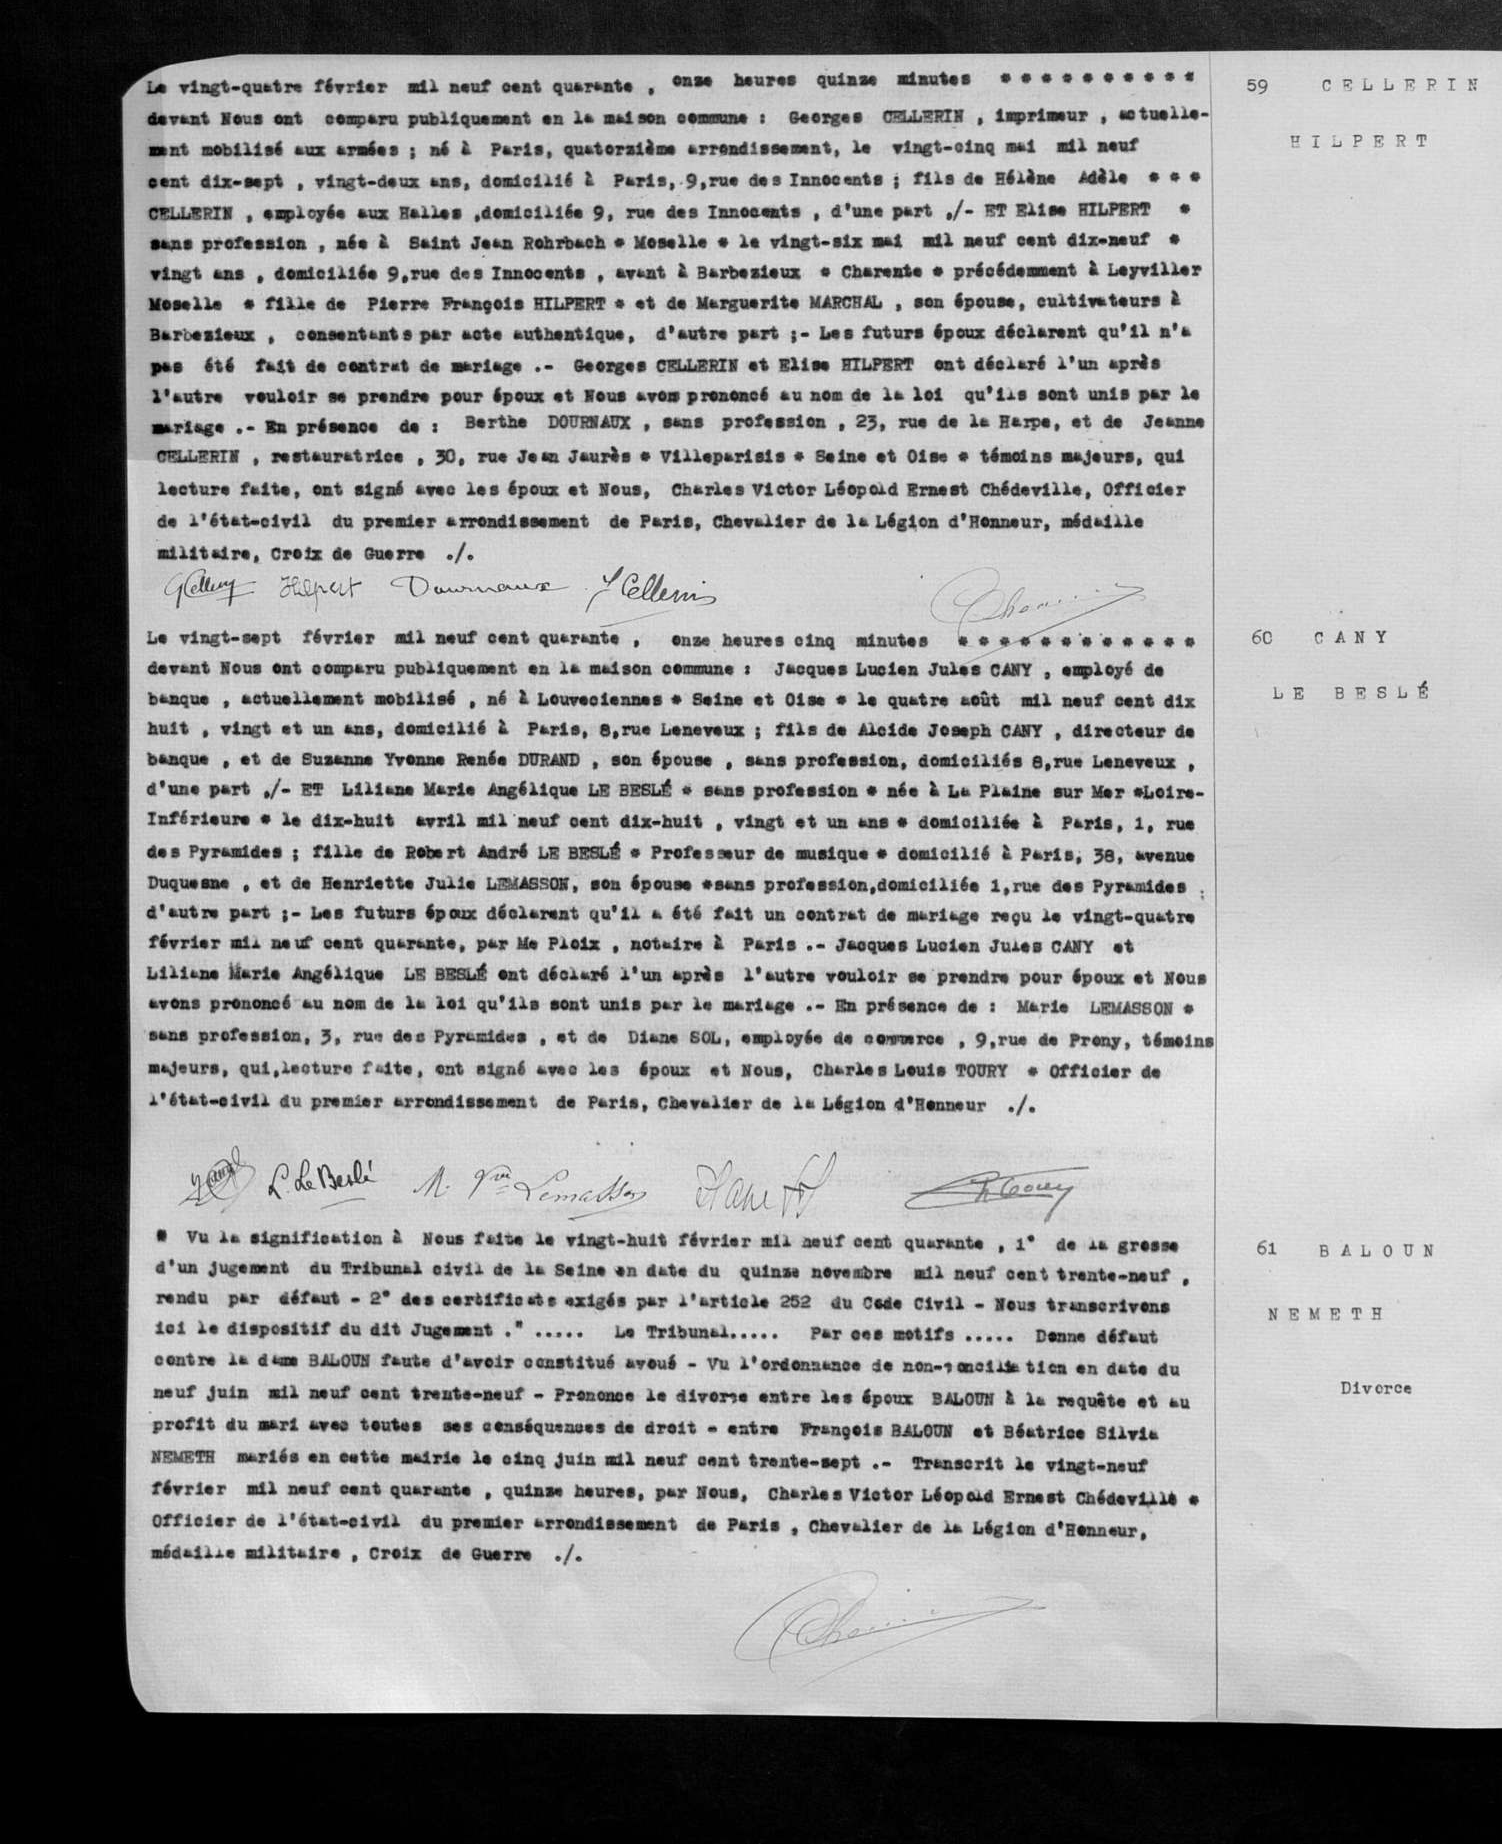Le vingt-quatre février mil neuf cent quarante, onze heures quinze minutes ****
devant Nous ont comparu publiquement en la maison commune: Georges CELLERIN, imprimeur, actuelle-
ment mobilisé aux armées; né à Paris, quatorzième arrondissement, le vingt-cinq mai mil neuf
cent dix-sept, vingt-deux ans, domicilié à Paris, 9, rue des Innocents; fils de Hélène Adèle ***
CELLERIN, employée aux Halles, domiciliée 9, rue des Innocents, d'une part ,/-ET Elise HILPERT *
sans profession, née à Saint Jean Rohrbach * Moselle * le vingt-six mai mil neuf cent dix-neuf *
vingt ans, domiciliée 9, rue des Innocents, avant à Barbezieux * Charente * précédemment à Leyviller
Moselle * fille de Pierre François HILPERT * et de Marguerite MARCHAL, son épouse, cultivateurs à
Barbesieux, consentants par acte authentique, d'autre part ;-Les futurs époux déclarent qu'il n'a
pas été fait de contrat de mariage .-George CELLERIN et Elise HILPERT ont déclaré l'un après
l'autre vouloir se prendre pour époux et Nous avons prononcé au nom de la loi qu'ils sont unis par le
mariage .-En présence de: Berthe DOURNAUX, sans profession, 23, rue de la Harpe, et de Jeanne
CELLERIN, restauratrice, 30, rue Jean Jaurès * Villeparisis * Seine et Oise * témoins majeurs, qui
lecture faite, ont signé avec les époux et Nous, Charles Victor Léopold Ernest Chédeville, Officier
de l'état-civil du premier arrondissement de Paris, Chevalier de la Légion d'Honneur, médaille
militaire, Croix de Guerre ./.
Le vingt-sept février mil neuf cent quarante, onze heures cinq minutes ****
devant Nous ont comparu publiquement en la maison commune: Jacques Lucien Jules CANY, employé de
banque, actuellement mobilisé, né à Louveciennes * Seine et Oise * le quatre août mil neuf cent dix
huit, vingt et un ans, domicilié à Paris, 8, rue Leneveux; fils de Alcide Joseph CANY, directeur de
banque, et de Suzanne Yvonne Renée DURAND, son épouse, sans professions, domiciliés 8, rue Leneveux,
d'une part ,/-ET Liliane Marie Angélique LE BESLE * sans profession * née à La Plaine sur Mer * Loire-
Inférieure * le dix-huit avril mil neuf cent dix-huit, vingt et un ans * domiciliée à Paris, 1, rue
des Pyramides; fille de Robert André LE BESLE * Professeur de musique * domicilié à Paris, 38, avenue
Duquesne, et de Henriette Julie LEMASSON, son épouse * sans profession, domiciliée 1, rue des Pyramides
d'autre part ;-Les futurs époux déclarent qu'il a été fait un contrat de mariage reçu le vingt-quatre
février mil neuf cent quarante, par Me Ploix, notaire à Paris .-Jacques Lucien Jules CANY et
Liliane Marie Angélique LE BESLE ont déclaré l'un après l'autre vouloir se prendre pou époux et Nous
avons prononcé au nom de la loi qu'ils sont unis par le mariage .-En présence de: Marie LEMASSON *
sans profession, 3, rue des Pyramides, et de Diane SOL, employée de commerce, 9, rue de Prony, témoins
majeurs, qui, lecture faite, ont signé avec les époux et Nous, Charles Louis TOURY * Officier de
l'état-civil du premier arrondissement de Paris, Chevalier de la Légion d'Honneur ./.
Vu la signification à Nous faite le vingt-huit février mil neuf cent quarante, 1° de la grosse
d'un jugement du Tribunal civil de la Seine en date du quinze novembre mil neuf cent trente-neuf,
rendu par défaut-2° des certificats exigés par l'article 252 du Code Civil-Nous transcrivons
ici le dispositif du dit Jugement ." .... Le Tribunal.... Par ces motifs..... Donne défaut
contre la dame BALOUN faute d'avoir constitué avoué-Vu l'ordonnance de non-conciliation en date du
neuf juin mil neuf cent trente-neuf-Prononce le divorce entre les époux BALOUN à la requête et au
profit du mari avec toutes ses conséquences de droit-entre François BALOUN et Béatrice Silvia
NEMETH mariés en cette mairie le cinq juin mil neuf cent trente-sept .-Transcrit le vingt-neuf
février mil neuf cent quarante, quinze heures, par Nous, Charles Victor Léopold Ernest Chédeville *
Officier de l'état-civil du premier arrondissement de Paris, Chevalier de la Légion d'Honneur,
médaille militaire, Croix de Guerre ./.
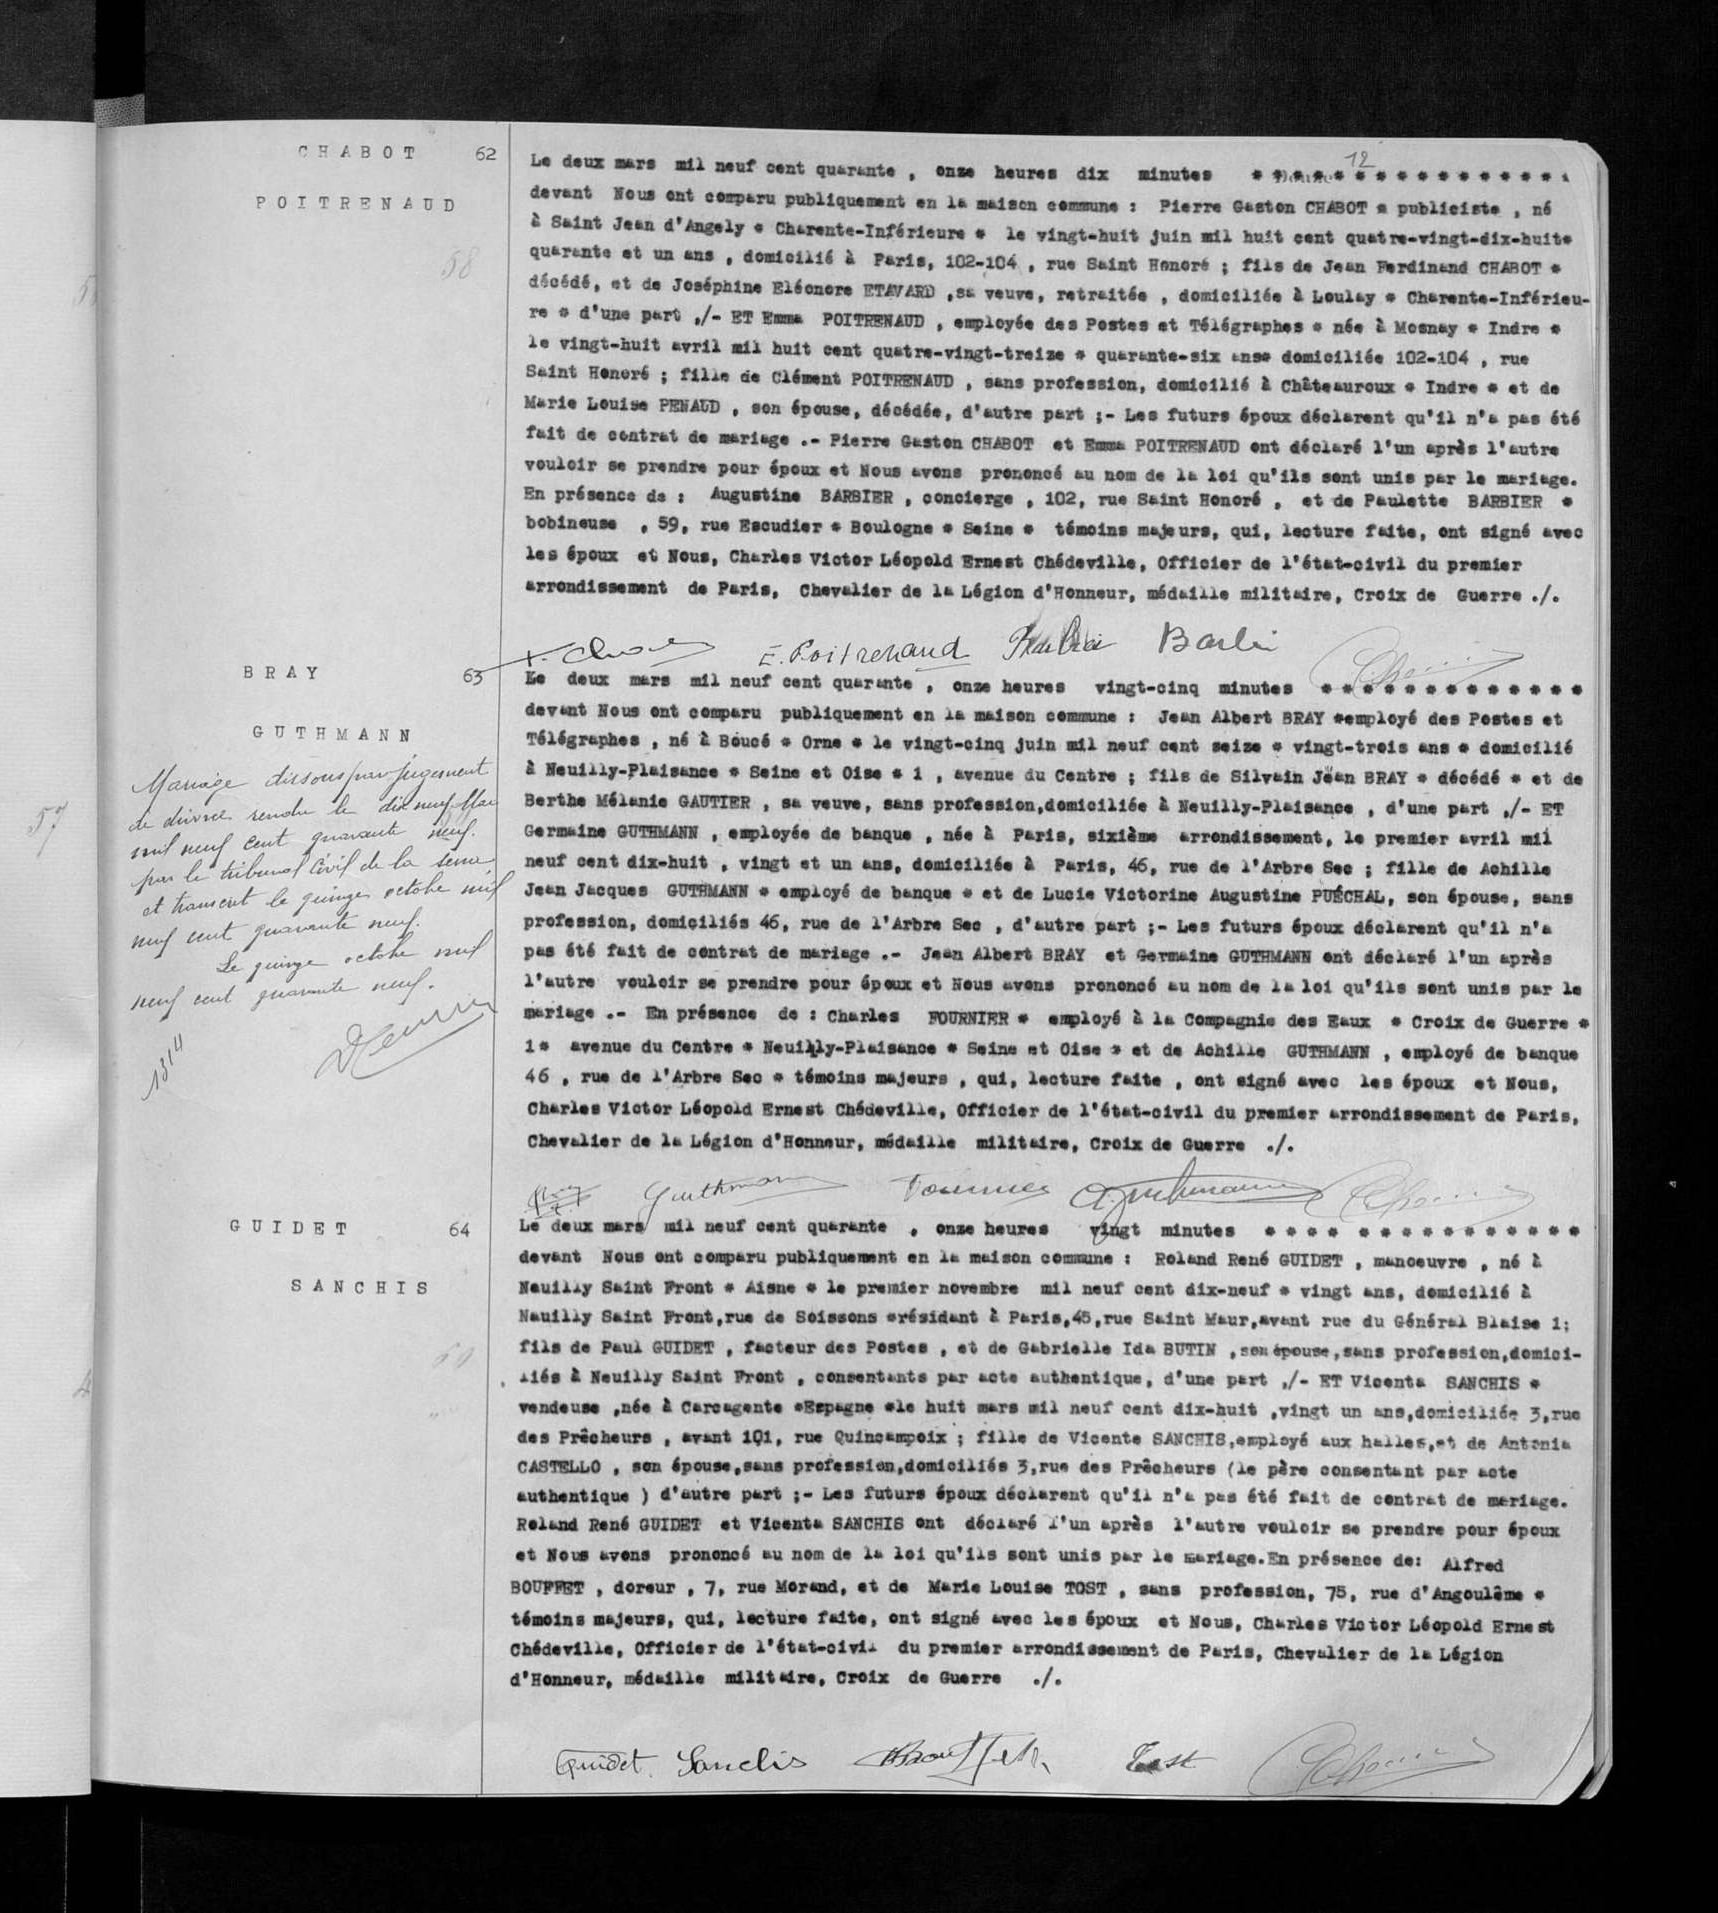Le deux mars mil neuf cent quarante, onze heures dix minutes ****
devant Nous ont comparu publiquement en la maison commune: Pierre Gaston CHABOT * publiciste, né
à Saint Jean d'Angle * Charente-Inférieure * le vingt-huit juin mil huit cent quatre-vingt-dix-huit
* quarante et un ans, domicilié à Paris, 102-104, rue Saint Honoré; fils de Jean Ferdinand CHABOT *
décédé, et de Joséphine Eléonor ETAVARD, sa veuve, retraitée, domiciliée à Loulay * Charente-Inférieu-
re * d'une part ,/-ET Emma POITRENAUD, employée des Postes et Télégraphes * né à Mosnay * Indre *
le vingt-huit avril mil huit cent quatre-vingt-treize * quarante-six ans * domiciliée 102-104, rue
Saint Honoré; fille de Clément POITRENAUD, sans profession, domicilié à Châteauroux * Indre * et de
Marie Louise PENAUD, son épouse, décédée, d'autre part ;-Les futurs époux déclarent qu'il n'a pas été
fait de contrat de mariage .-Pierre Gaston CHABOT et Emma POITRENAUD ont déclaré l'un après l'autre
vouloir se prendre pour époux et Nous avons prononcé au nom de la loi qu'ils sont unis par le mariage.
En présence de Augustin BARBIER, concierge, 102, rue Saint Honoré, et de Paulette BARBIER *
bobineuse, 59, rue Escudier * Boulogne * Seine * témoins majeurs, qui, lecture faite, ont signé avec
les époux et Nous, Charles Victor Léopold Ernest Chédeville, Officier de l'état-civil du premier
arrondissement de Paris, Chevalier de la Légion d'Honneur, médaille militaire, Croix de Guerre ./.
Le deux mars mil neuf cent quarante, onze heures vingt-cinq minutes ****
devant Nous ont comparu publiquement en la maison commune: Jean Albert BRAY * employé des Postes et
Télégraphes, né à Boucé * Orne * le vingt-cinq juin mil neuf cent seize * vingt-trois ans * domicilié
à Neuilly-Plaisance * Seine et Oise * 1, avenue du Centre; fils de Silvain Jean BRAY * décédé * et de
Berthe Mélanie GAUTIER, sa veuve, sans profession, domiciliée à Neuilly-Plaisance, d'une part ,/-ET
Germaine GUTHMANN, employée de banque, née à Paris, sixième arrondissement, le premier avril mil
neuf cent dix-huit, vingt et un ans, domicilié à Paris, 46, rue de l'Arbre Sec; fille de Achille
Jean Jacques GUTHMANN * employé de banque * et de Lucie Victorine Augustine PUECHAL, son épouse, sans
profession, domiciliés 46, rue de l'Arbre Sec, d'autre parts ;-Les futurs époux déclarent qu'il n'a
pas été fait de contrat de mariage .-Jean Albert BRAY et Germaine GUTHMANN ont déclaré l'un après
l'autre vouloir se prendre pour époux et Nous avons prononcé au nom de la loi qu'ils sont unis par le
mariage .-En présence de: Charles FOURNIER * employé à la Compagnie des Eaux * Croix de Guerre *
1 * avenue du Centre * Neuilly-Plaisance * Seine et Oise * et de Achille GUTHMANN, employé de banque
46, rue de l'Arbre Sec * témoins majeurs, qui, lecture faite, ont signé avec les époux et Nous,
Charles Victor Léopold Ernest Chédeville, Officier de l'état-civil du premier arrondissement de Paris,
Chevalier de la Légion d'Honneur, médaille militaire, Croix de Guerre ./.
Le deux mars mil neuf cent quarante, onze heures vingt minutes ****
devant Nous ont comparu publiquement en la maison commune: Roland René GUIDET, manoeuvre, né à
Neuilly Saint Front * Aisne * le premier novembre mil neuf cent dix-neuf * vingt ans, domicilié à
Neuilly Saint Front, rue de Soissons * résidant à Paris, 45, rue Saint maur, avant rue du Général Blaise 1;
fils de Paul GUIDET, facteur des Postes, et de Gabrille Ida BUTIN, son épouse, sans profession, domici-
liés à Neuilly Saint Front, consentants par acte authentique, d'une part ,/-ET Vicents SANCHIS *
vendeuse, née à Carcagente * Espagne * le huit mars mil neuf cent dix-huit, vingt un ans, domiciliée 3, rue
des Prêcheurs, avant 101, rue Quincampoix; fille de Vincente SANCHIS, employé aux halles, et de Antonia
CASTELLO, son épouse, sans profession, domiciliés 3, rue des Prêcheurs (le père consentant par acte
authentique) d'autre part ;-Les futurs époux déclarent qu'il n'a pas été fait de contrat de mariage.
Roland René GUIDET et Vincent SANCHIS ont déclaré l'un après l'autre vouloir se prendre pour époux
et Nous avons prononcé au nom de la loi qu'ils sont unis par le mariage. En présence de: Alfred
BOUFFET, doreur, 7, rue Morand, et de Marie Louis TOST, sans profession, 75, rue d'Angoulême *
témoins majeurs, qui, lecture faite, ont signé avec les époux et Nous, Charles Victor Léopold Ernest
Chédeville, Officier de l'état-civil du premier arrondissement de Paris, Chevalier de la Légion
d'Honneur, médaille militaire, Croix de Guerre ./.